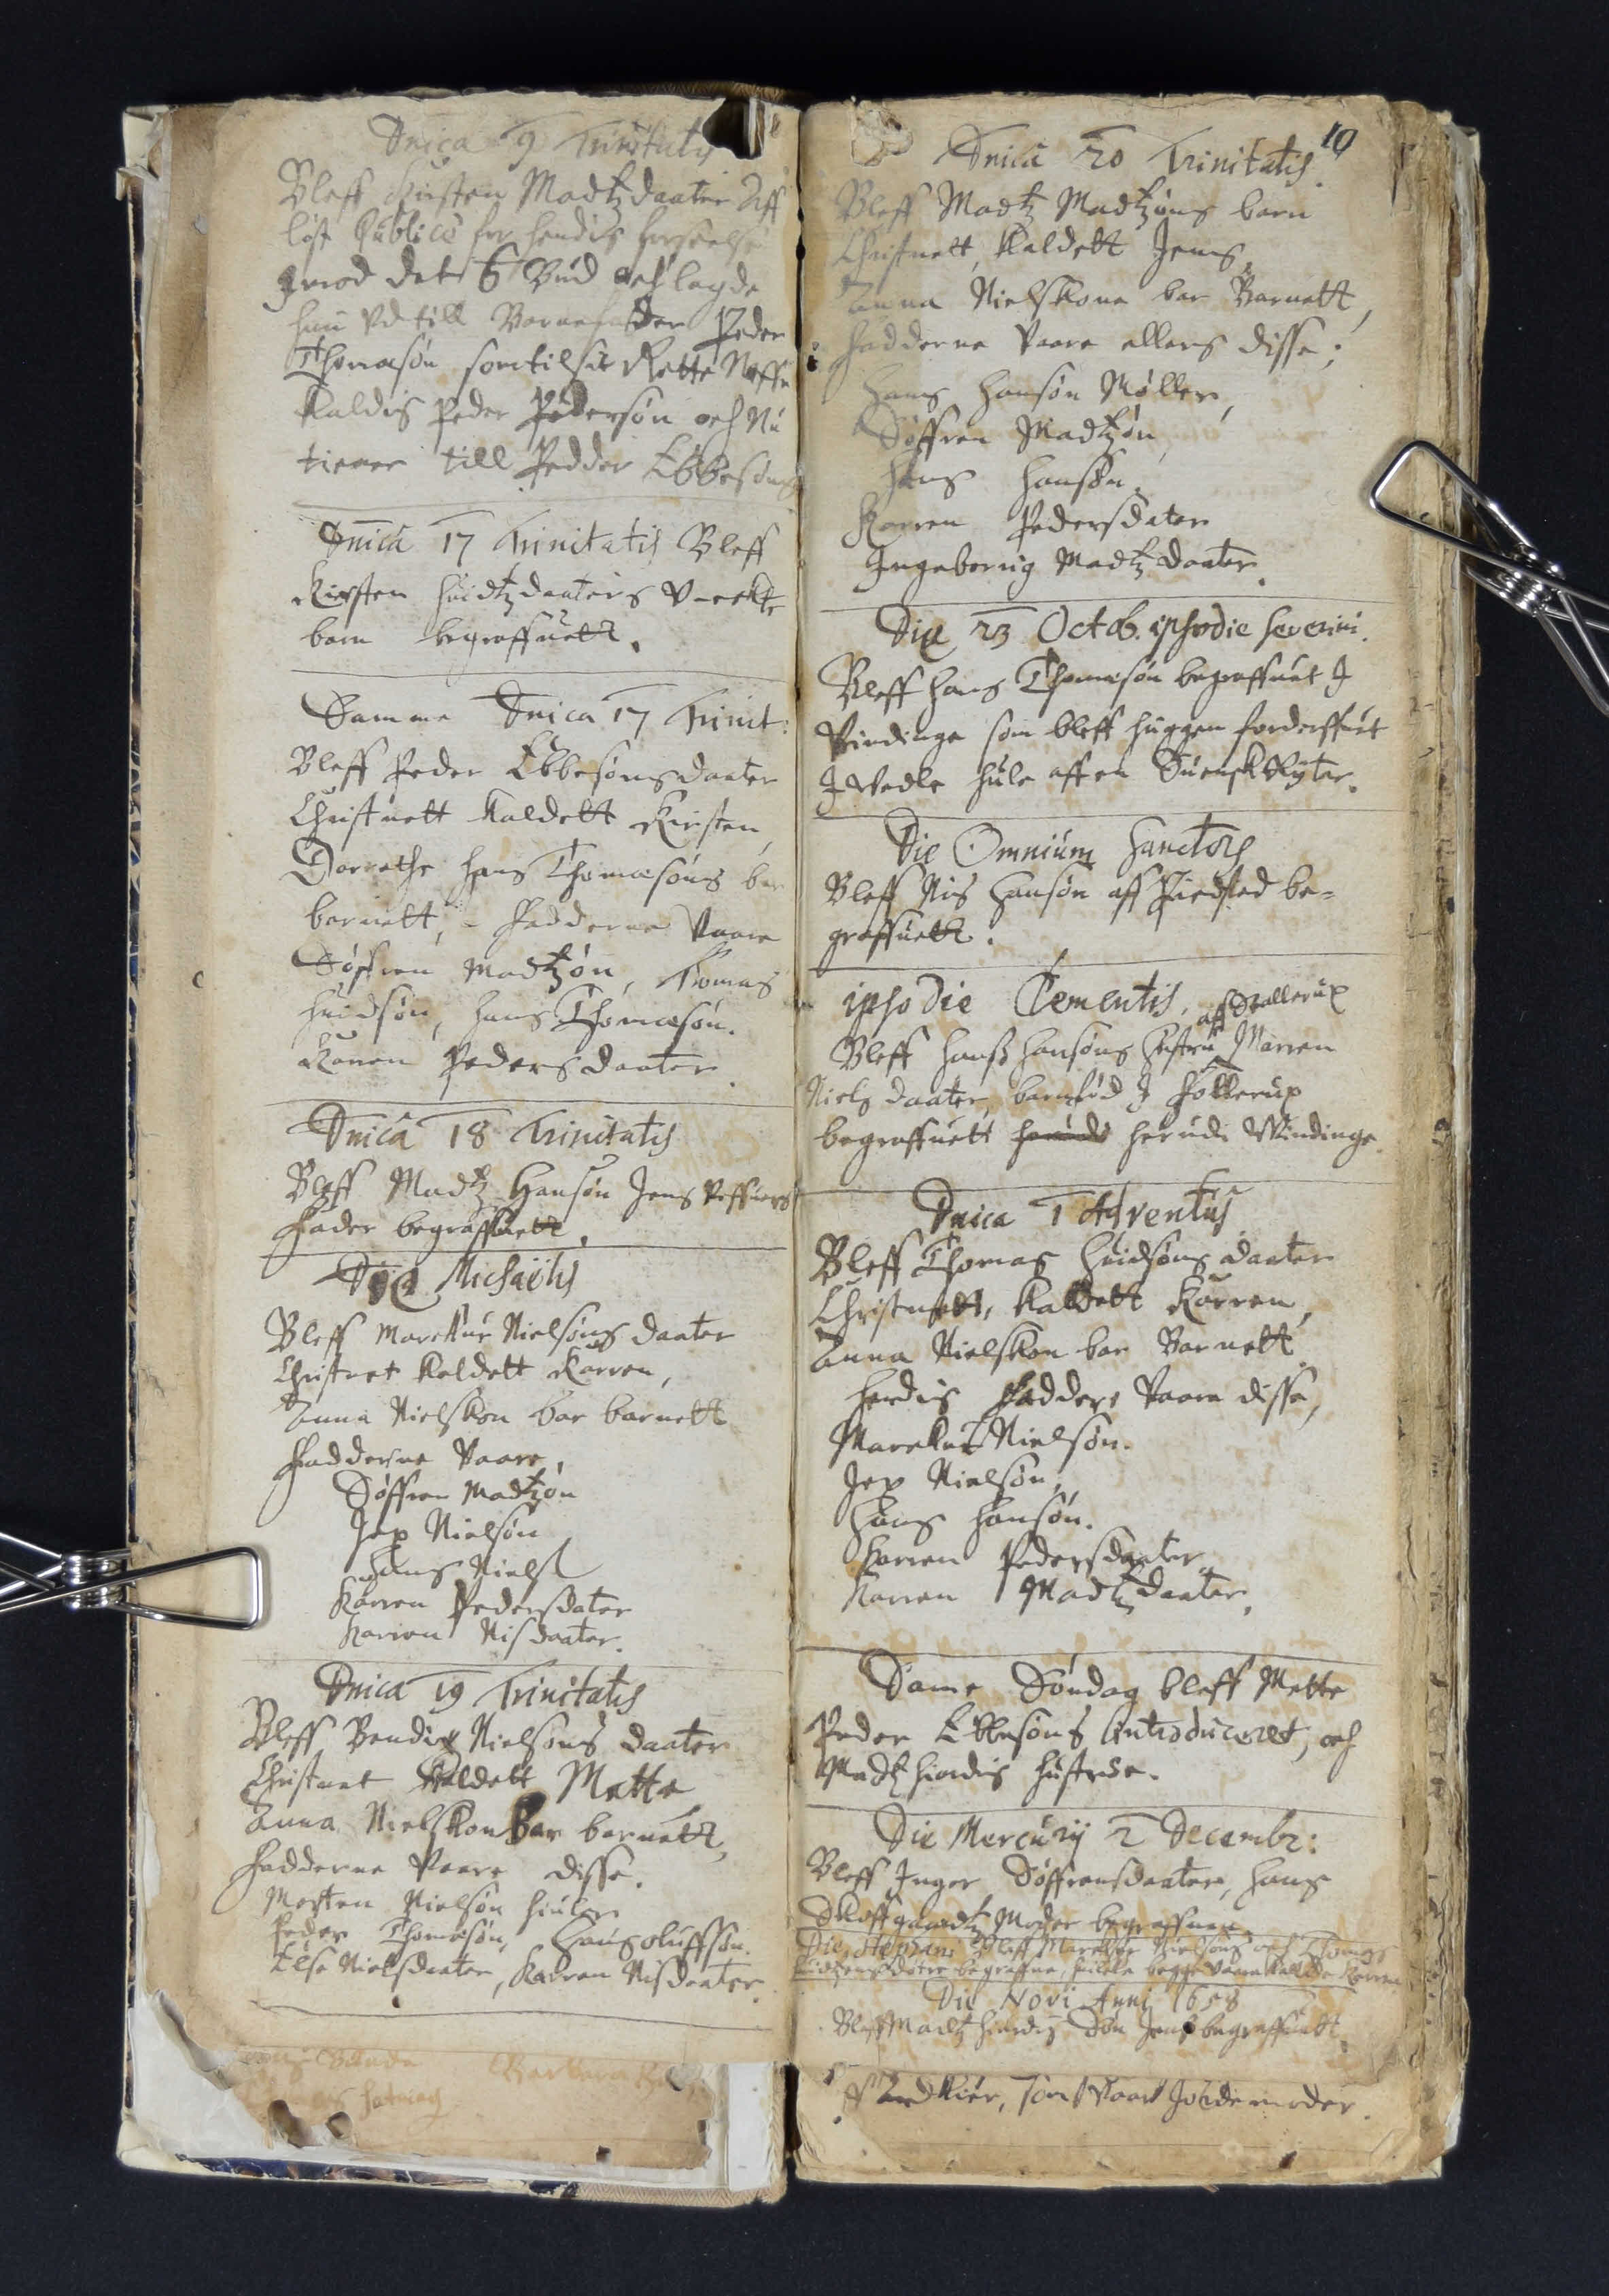Dnica 9 Trinitatis
Bleff Kirsten Madtzdaater Aff 
løft Publica for hendis forseelse 
I mod det 6 Bud och lagde
hun ud till Barnefader Peder 
Thomasøn, som tilsit Rette Naffn
kaldis Peder Pedersøn och Nu
tiener till Pedder Ebbesøns
Dnica 17 Trinitatis Bleff
Kiersten Hvidtz daaters V-eekte
barn begraffuett.
Samme Dnica 17 Trinit:
Bleff Peder Ebbesøns daater
Christnett kaldett Kisten
Dorrothe Hans Thomasøns bar
barnett, - Fadderne Vaare
Søfren Madtzøn. Thomas
Hvidsøn. Hans Thomasøn.
Karen Pedersdaater.
Dnica 18 Trinitatis
Bleff Madtz Hansøn Jens Veffuers
Fader begraffuett.
Dic Michaelis
Bleff Markus Nielsøns daater
Christnet kaldett Karren
Anna Niels Kon bar barnett
Fadderne Vaare
Søffren Madtzøn
Jep Nielsøn
Hans Niels
Karren Pedersdater
Karren Nisdaater.
Dnica 19 trinitatis
Bleff Bendix Nielsøns daater
Christnet kaldett Mette
Anna Niels Kon bar barnett
Fadderne Vaare disse.
Morten Nielsøn Hiuler
Peder Thomasøn. Hans Oluffsøn
Else Nielsdaater, Karren Nisdaater
10
Dnica 20 Trinitatis.
Bleff Madtz Madtzøns barn
Christnett, kaldett Jens,
Anna Niels Kone bar barnett
Fadderne Vaare ellers disse
Hans Hansøn Møller.
Søffren Madtzøn
Hans Hansøn
Karren Pedersdater.
Ingeborig Madtzdaater.
Dica 23 Octob. ##schordie scavenius##
Bleff Hans Thomasøn begraffuet I
Vindinge som bleff huggen forderffuet
I Wedle hule aff en Suensk Ryter.
Die Omnium Sanctore
Bleff Nis Hansøn aff Piedsted be¬
graffuett.
ipso die Clementis.
af Stallerup
Bleff Hanss Hansøns Hhustru Marren
Niels daater barnfød I Follerup
begraffuett herudi her udi Windinge.
Dnica 1 Adventus
Bleff Thomas Hvidsøns daater
Christnett, kaldett Karren,
Anna Niels Kon bar Barnett
hendis Faddere vaare disse,
Marckus Nielsøn.
Jep Nielsøn
Hans Hansøn.
Karren Pedersdaater.
Karren Madtzdaater.
Same Søndag bleff Mette
Peder Ebbesøns Introduceret och
Madtz Hiordis hustrue.
Die Mercurij 2 Decembr:
Bleff Inger Søffrensdaater, Hans
Skoffgaardtz Moder begraffuen
Die Stephani Bleff Marckus Nielsøns och Thomas
Hvidtzøns døtre begraffne, huilcke begge vaare kaldet Karren
Die Novi Anni 1658
Bleff Madtz Hiurdis Søn Jens begraffuett
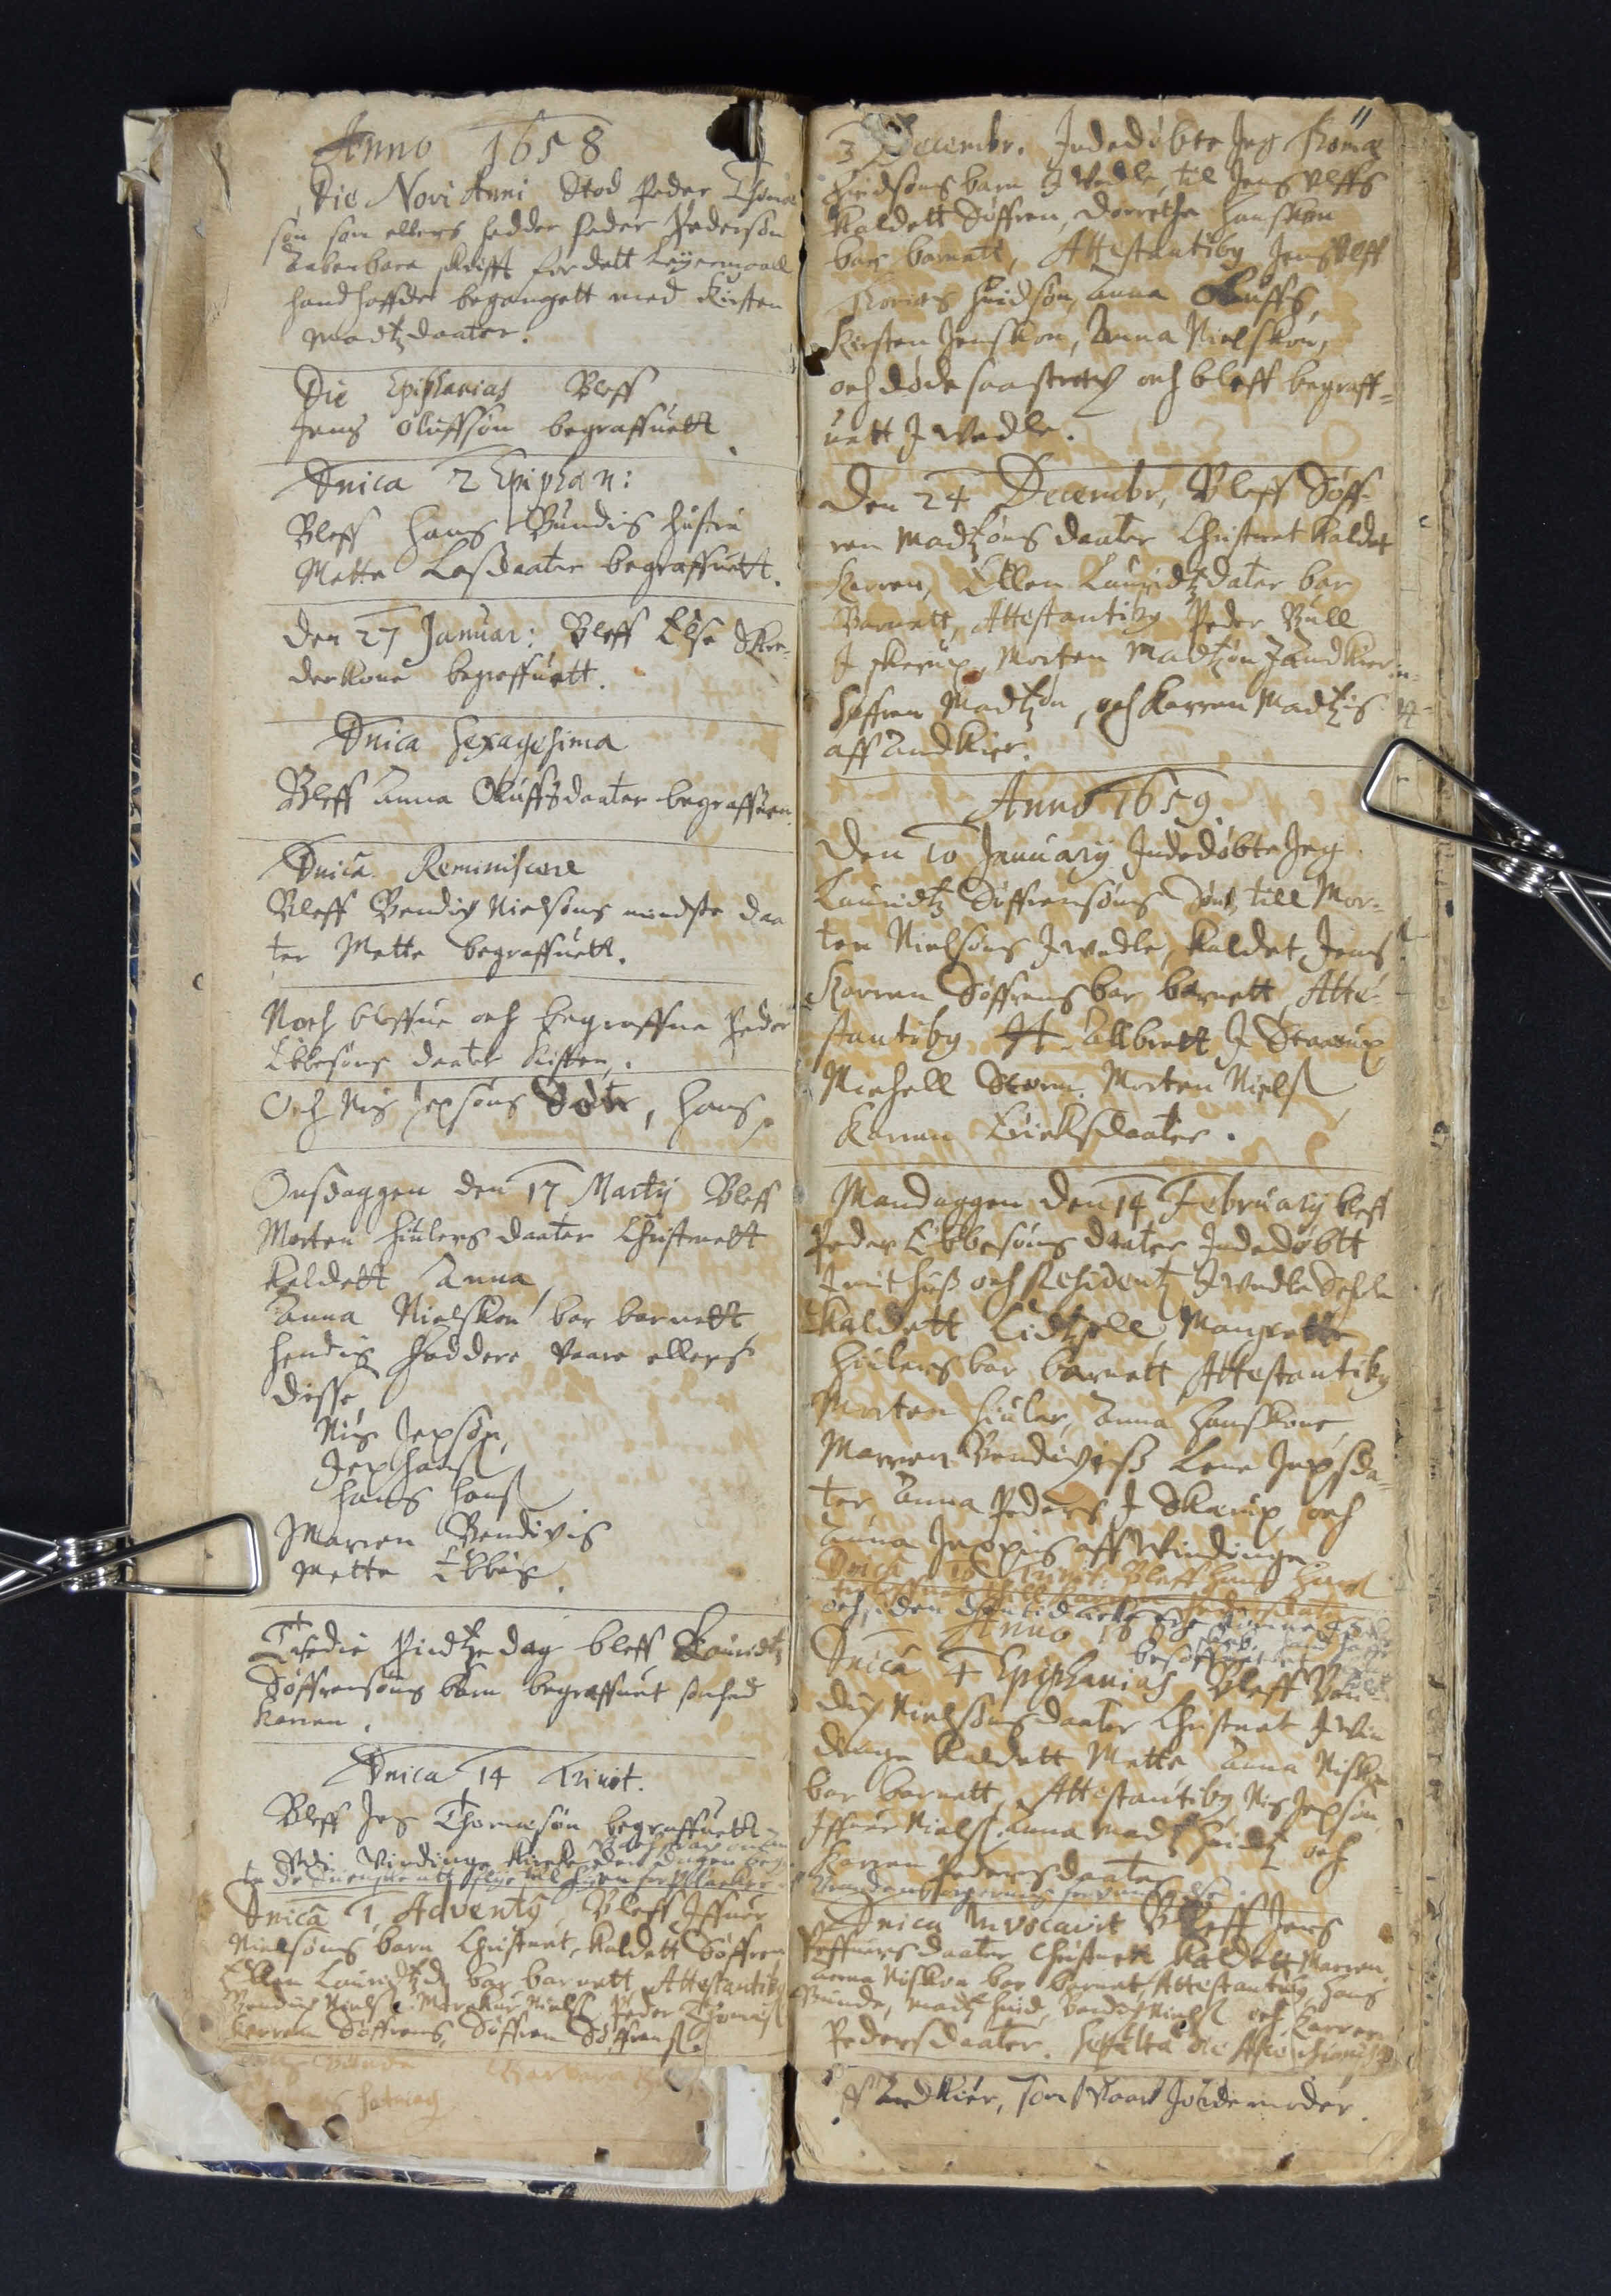Anno 1658
Die Novi Anni. Stod Peder Thoma
søn som ellers hedder Peder Pedersøn 
Aabenbare skrifft for dett leyermaal
hand haffde begangett med Kirsten 
Madtzdaater
Die Epiphanias Bleff
Hans Oluffsøn begraffuett
Dnica 2 Epiphan:
Bleff Hans Bundis hustru
Mette Lassdaater begraffuett
Den 27 Januar: Bleff Else Skre¬
der Kone begraffuett
Dnica Sexagesima
Bleff Anna Oluffsdaater begraffuen
Dnica Reminiscere
Bleff Bendix Nielsøns mindste daa
ter Mette begraffuett
Noch bleffue och begraffue Peder
Ebbesøns daater Kisten
Och Nis Jepsøns Søn, Hans
Onsdaggen den 17 Martij Bleff
Morten Hiulers daater Christnett
kaldett Anna
Anna Niels Kon bar barnett
Hendis faddere vaare ellers
disse
Nis Jepsøn
Jep Hans
Hans Hans
Marren Bendixis
Mette Ebbis
Tredie Pindtze dag. bleff Lauridtz
Søffrensøns barn begraffuet som hed 
Karren
Dnica 14 Trinit.
Bleff Jes Thomasøn begraffuett
NB och strax
Vdi Vindinge Kircke. den dagen beg 
te de Suenske att flye till Fuen for Polacker
Dnica 1 Adventq, Bleff Iffuer
Nielsøns barn Christnet, kaldett Søffren
Ellen Lauritzd bar barnett, Attestantiby
Bendix Niels Markus Niels Peder Thomas
Karren Søffrens, Søffren Søffrens.
11
3 Decembr. Indedøbte Jeg Thomas
Hvidsøns barn I Vedle, til Jens Vlffs,
kaldett Søffren, Dorothe Hans kon
bar barnet, Attestantiby Jens Vlff
Thomas Hvidsøn, Anna Oluffs.
Kirsten Jens Kon, Anna Niels Kon,
och døde saa strax och bleff begraff¬
uett I Wedle.
Den 24 Decembr, Bleff Søff¬
ren Madtzøns daater Christnet, kaldet
Karren, Ellen Lauritzdater bar
Barnett. Attestantiby Peder Bull
I Skerup. Morten Madtzøn I Andkier
Søffren Madtzøn och Karren Madtzis
aff Andkier
Anno 1659.
den 10 Januarij. Indedøbte Jeg 
Lauridtz Søffrensøns Søn till Mor¬
ten Nielsøns I Wedle, kaldet JENS
Karren Søffrens bar barnet. Atte¬
stantiby Hl## Allbertt I Staarup
Michell Skom. Morten Niels
Karren Ericksdaater.
Mandaggen den 14 Februarij bleff
Peder Ebbesøns daater Indedøbt
I mit Huss och Residentz I Wedle Schole
kaldett Cidtzell, Margrette
Hiulers bar barnet. Attestantiby
Morten Hiuler, Anna Hans Kone
Marren Bendixiss Lene Jepsda¬
ter. Anna Peders I Skarup och
Anna Jeppis aff Windinge.
Dnica 16 Trinit: Bleff Hans Hans
troloffuet thill Karren Pedersdater
och siden den tid ## ## ## ##
## ##
besoffuet ##
Anno 1660
Dnica 4 Epiphanias Bleff Ben¬
dix Nielsøns daater Christnet I Win
dinge, kaldett Mette Anna Nis
bar barnett. Attestantiby Nis Jepsøn
Iffuer Niels. Anna Madtz Hvidtz och
Karren Pedersdaater.
##
Dnica Invocavit Bleff jens
Veffuers daater christnett kaldett Marren.
Anna Nis Kon bar barnet, Attistantiby, Hans
bunde, Madtz Hvid, Bendix Niels och Karren 
Pedersdaater. Sepultra ## Asscionsionis##